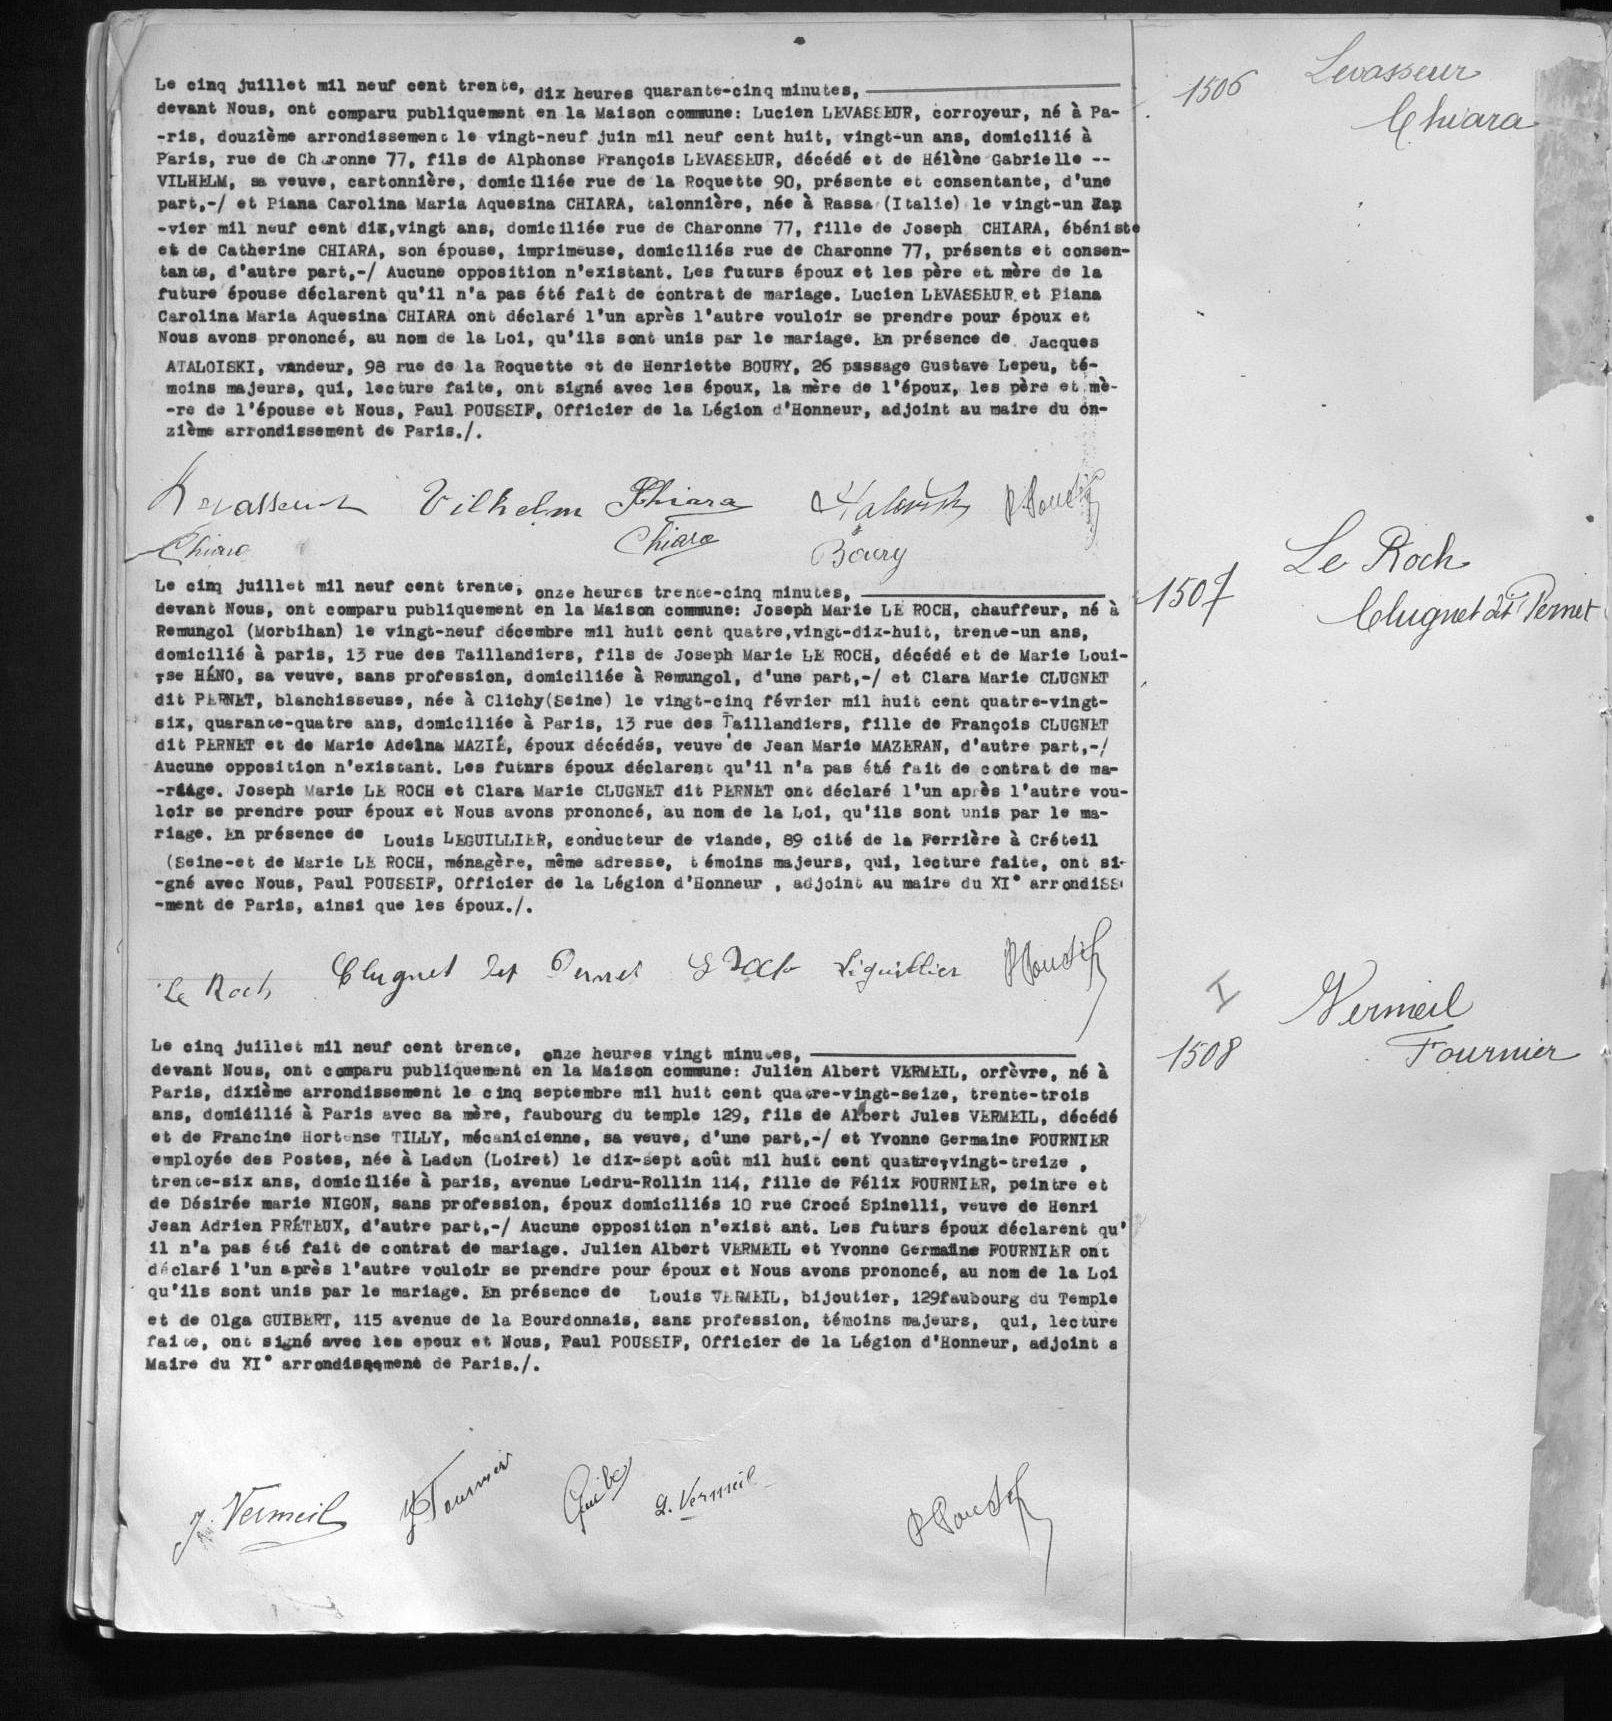Le cinq juillet mil neuf cent trente, dix heures quarante-cinq minutes, -
devant Nous, ont comparu en la Maison commune: Lucien LEVASSEUR, corroyeur, né à Pa-
-ris, douzième arrondissement le vingt-neuf juin mil neuf cent huit, vingt-un ans, domicilié à
Paris, rue de Charonne 77, fils de Alphonse François LEVASSEUR, décédé et de Hélène Gabrielle -
VILHELM, sa veuve, cartonnière, domiciliée rue de la Roquette 90, présente et consentante, d'une
part ,-/ et Piana Carolina Maria Aquesina CHIARA, talonnière, née à Rassa (Italie) le vingt-un Van
-vier mil neuf cent dix, vingt ans, domiciliée rue de Charonne 77, fille de Joseph CHIARA, ébéniste
et de Catherine CHIARA, son épouse, imprimeuse, domiciliés rue de Charonne 77, présents et conse-
tants, d'autre part ,-/ Aucune opposition n'existant. Les futurs époux et les père et mère de la
future épouse déclarent qu'il n'a pas été fait de contrat de mariage. Lucien LEVASSEUR et Piana
Carolina Maria Aquesina CHIARA ont déclaré l'un après l'autre vouloir se prendre pour époux et
Nous avons prononcé, au nom de la Loi, qu'ils sont unis par le mariage. En présence de Jacques
ATALOISKI, vandeur, 98 rue de la Roquette et de Henriette BOURY, 26 passage Gustave Lepeu, té-
moins majeurs, qui, lecture faite, ont signé avec les époux, la mère de l'époux, les père et mè-
-re de l'épouse de Nous, Paul POUSSIF, Officier de la Légion d'Honneur, adjoint au maire du on-
zième arrondissement de Paris.
Le cinq juillet mil neuf cent trente, onze heures trente-cinq minutes ,-
devant Nous, ont comparu publiquement en la Maison commune: Joseph Marie LE ROCH, chauffeur, né à
Remungol (Morbihan) le vingt-neuf décembre mil huit cent quatre, vingt-dix-huit, trente-un ans,
domicilié à paris, 13 rue des Taillandiers, fils de Joseph Marie LE ROCH, décédé et de Marie Loui-
-se HENO, sa veuve, sans profession, domiciliée à Remungol, d'une part ,-/ et Clara Marie CLUGNET
dit PERNET, blanchisseuse, né à Clichy (Seine) le vingt-cinq février mil huit cent quatre-vingt-
six, quarante-quatre ans, domiciliée à Paris, 13 rue des Taillandiers, fille de François CLUGNET
dit PERNET et de Marie Adelna MAZIE, époux décédés, veuve de Jean Marie MAZERAN, d'autre part ,-/
Aucune opposition n'existant. Les futurs époux déclarent qu'il n'a pas été fait de contrat de ma-
-raage. Joseph Marie LE ROCH et Clara Marie CLUGNET dit PERNET ont déclaré l'un après l'autre vou-
loir se prendre pour époux et Nous avons prononcé, au nom de la Loi, qu'ils sont unis par le ma-
-riage. En présence de Louis LEGUILLIER, conducteur de viande, 89 cité de la Ferrière à créteil
(Seine-et de Marie LE ROCH, ménagère, même adresse, témoins majeurs, qui, lecture faite, ont si-
-gné avec Nous, Paul POUSSIF, Officier de la Légion d'Honneur, adjoint au maire du XI ° arrondiSS
-ment de Paris, ainsi que les époux ./.
Le cinq juillet mil neuf cent trente, onze heures vingt minutes, -
devant Nous, ont comparu publiquement en la Maison commune: Julien Albert VERMEIL, orfèvre, né à
Paris, dixième arrondissement le cinq septembre mil huit cent quatre-vingt-seize, trente-trois
ans, domicilié à Paris avec sa mère, aubourg du temple 129, fils de Albert Jules VERMEIL, décédé
et de Francine Hortense TILLY, mécanicienne, sa veuve, d'une part ,-/ et Yvonne Germaine FOURNIER
employée des Postes, née à Ladon (Loiret) le dix-sept août mil huit cent quatre-vingt-treize,
trente-six ans, domiciliée à paris, avenue Ledru-Rollin 114, fille de Félix FOURNIER, peintre et
de Désiré marie NIGON, sans profession, époux domiciliés 10 rue Crocé Spinelli, veuve de Henri
Jean Adrien PRETEUX, d'autre part ,-/ Aucune opposition n'existant. Les futurs époux déclarent qu'
il n'a pas été fait de contrat de mariage. Julien Albert VERMEIL et Yvonne Germaine FOURNIER ont
déclaré l'un après l'autre vouloir se prendre pour époux et Nous avons prononcé, au nom de la Loi
qu'ils sont unis par le mariage. En présence de Louis VERMEIL, bijoutier, 129 faubourg du Temple
et de Olga GUILBERT, 115 avenue de la Bourdonnais, sans profession, témoins majeurs, qui, lecture
faite, ont signé avec les epoux et Nous, Paul POUSSIF, Officier de la Légion d'Honneur, adjoint a
Maire du XI ° arrondissement de Paris ./.
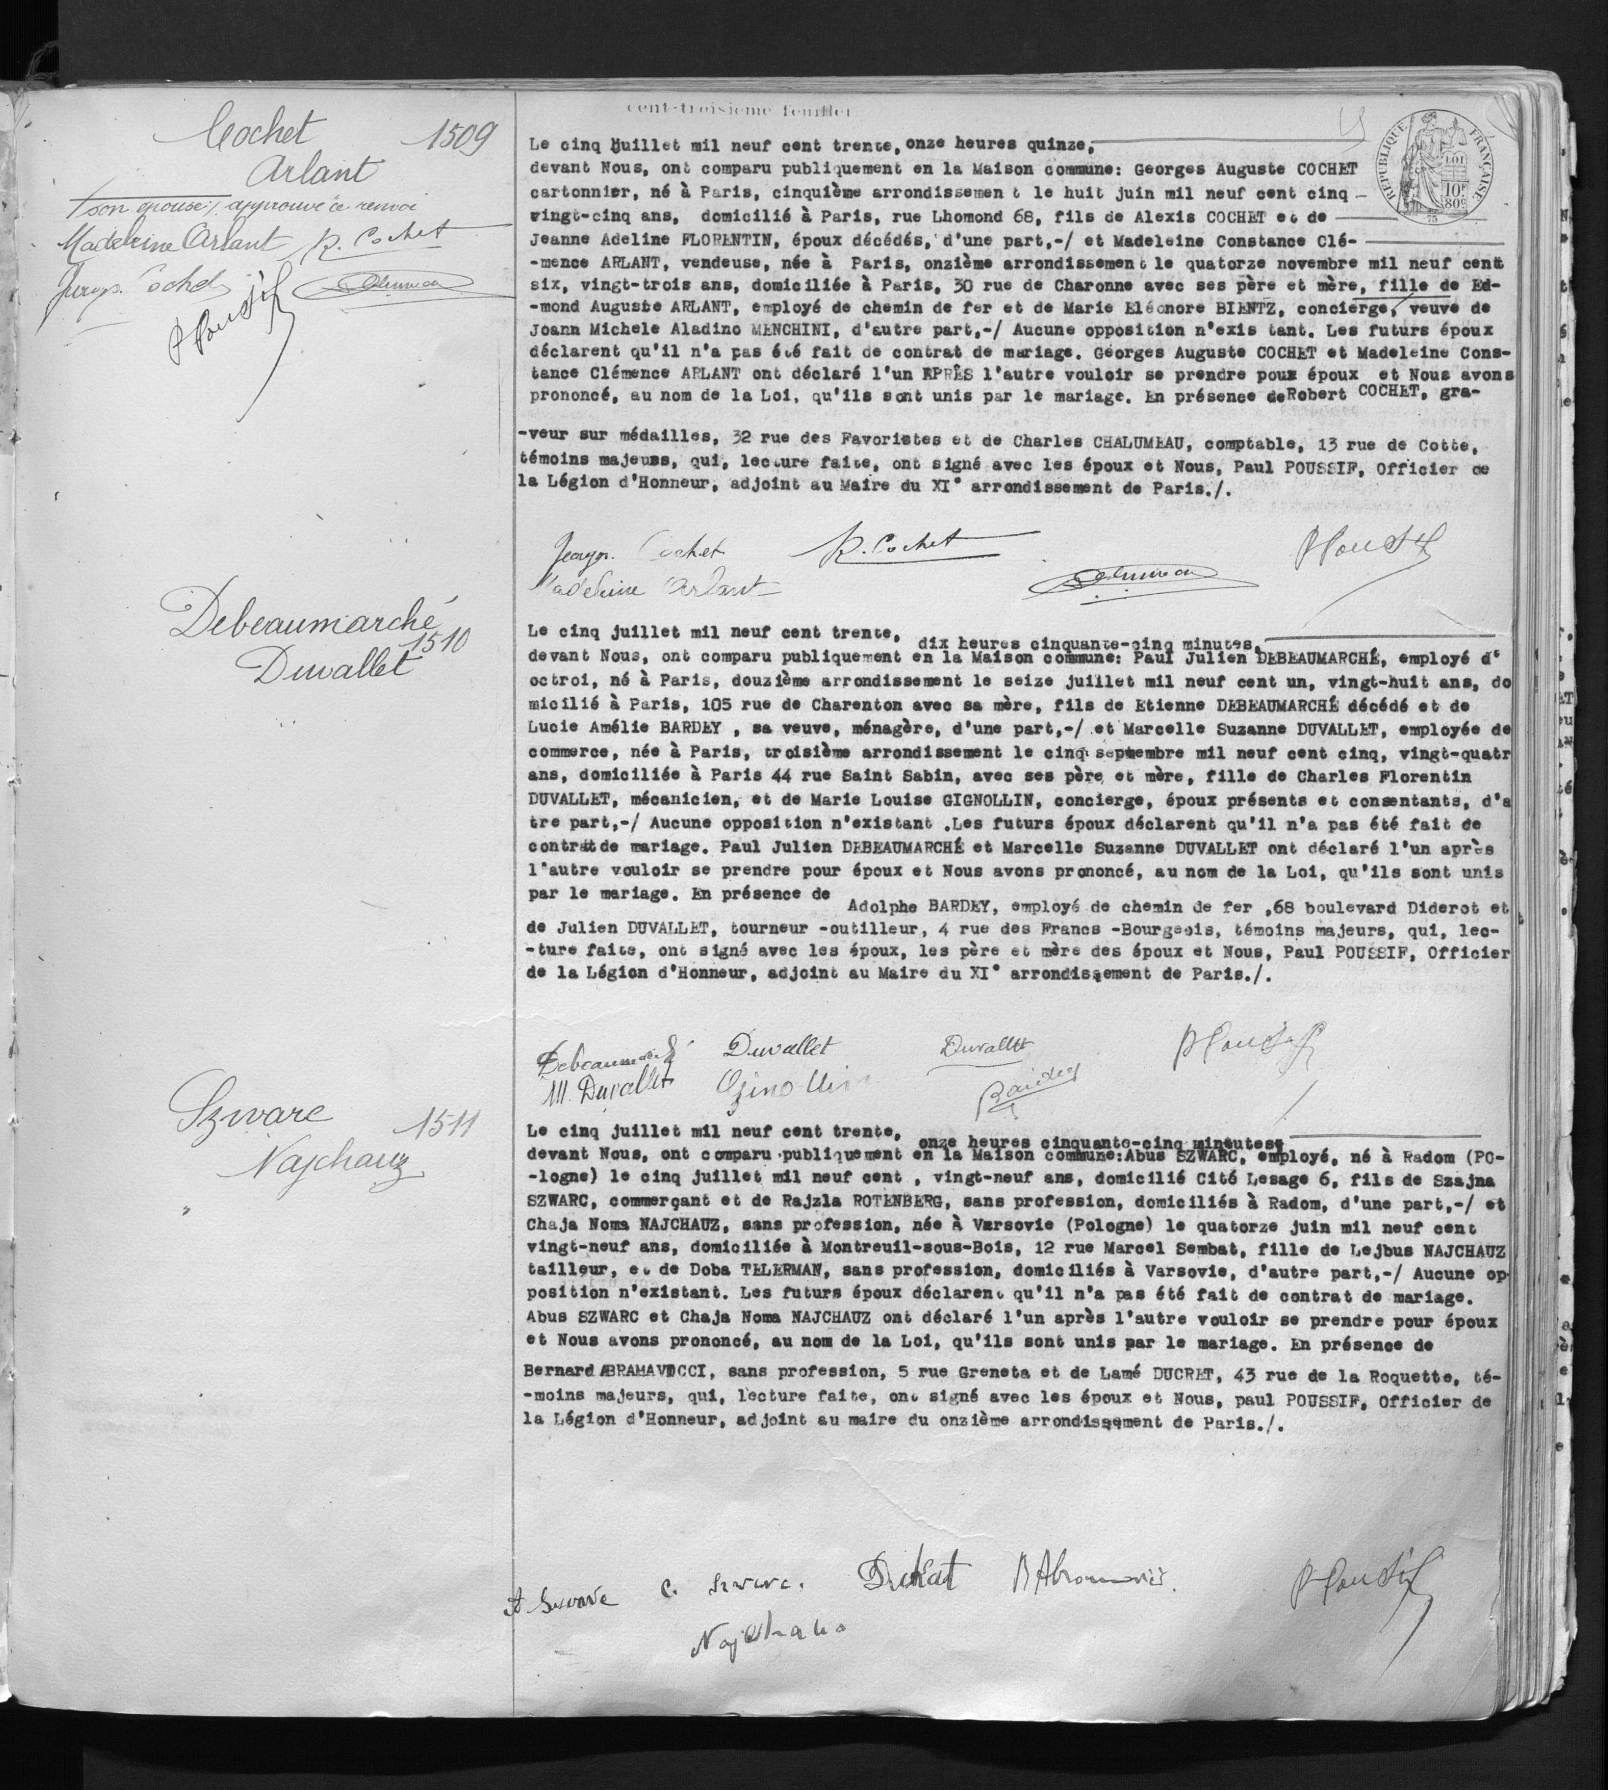Le cinq juillet mil neuf cent trente, onze heures quinze, -
devant Nous, ont comparu publiquement en la Maison commune: Georges Auguste COCHET
cartonnier, né à Paris, cinquième arrondissemen t le huit juin mil neuf cent cinq-
vingt-cinq ans, domicilié à Paris, rue Lhomond 68, fils de Alexis COCHET et de -
Jeanne Adeline FLORENTIN, époux décédés, d'une part ,-/ et Madeleine Constance Clé -
-mence ARLANT, vendeuse, née à Paris, onzième arrondissemen t le quatorze novembre mil neuf cent
six, vingt-trois ans, domiciliée à Paris, 30 rue de Charonne avec ses père et mère, fille de Ed-
-mond Auguste ARLANT, employé de chemin de fer et de Marie Eléonore BIENTZ, concierge, veuve de
Joann Michel Aladino MENCHINI, d'autre part ,-/ Aucune opposition n'exis tant. Les futurs époux
déclarent qu'il n'a pas été fait de contrat de mariage. Georges Auguste COCHET et Madelein Cons-
tance Clémence ARLANT ont déclaré l'un APRES l'autre vouloir se prendre pour époux et Nous avons
prononcé, au nom de la Loi, qu'ils sont unis par le mariage. En présence de Robert COCHET, gra-
-veur sur médailles, 32 rue des Favoristes et de Charles CHALUMEAU, comptable, 13 rue de Cotte,
témoins majeurs, qui, lecture faite, ont signé avec les époux et Nous, Paul POUSSIF, Officier ce
la Légion d'Honneur, adjoint au Maire du XI ° arrondissement de Paris.
Le cinq juillet mil neuf cent trente, dix heures cinquante-cinq minutes ,-
devant Nous, ont comparu publiquement en la Maison commune: Paul Julien DEBEAUMARCHE, employé d'
octroi, né à Paris, douzième arrondissement le seize juillet mil neuf cent un, vingt-huit ans, do
micilié à Paris, 105 rue de Charenton avec sa mère, fils de Etienne DEBEAUMARCHE décédé et de
Lucie Amélie BARDEY, sa veuve, ménagère, d'une part ,-/ et Marcelle Suzanne DUVALLET, employée de
commerce, née à Paris, troisième arrondissement le cinq septembre mil neuf cent cinq, vingt-quatr
ans, domiciliée à Paris 44 rue Saint Sabin, avec ses père et mère, fille de Charles Florentin
DUVALLET, mécanicien, et de Marie Louis GIGNOLLIN, concierge, époux présents et consentants, d'au
tre part ,-/ Aucune opposition n'existant. Les futurs époux déclarent qu'il n'a pas été fait de
contrat de mariage. Paul Julien DEBAUMARCHE et Mercelle Suzanne DUVALLET ont délcaré l'un après
l'autre vouloir se prendre pour époux et Nous avons prononcé, au nom de la Loi, qu'ils sont unis
par le mariage. En présence de Adolphe BARDEY, employé de chemin de fer, 68 boulevard Diderot et
de Julien DUVALLET, tourneur-outilleur, 4 rue des Francs-Bourgeois, témoins majeurs, qui, lec-
-ture faite, ont signé avec les époux, les père et mère des époux et Nous, Paul POUSSIF, Officier
de la Légion d'Honneur, adjoint au Maire du XI ° arrondissement de Paris ./.
Le cinq juillet mil neuf cent trente, onze heures cinquante-cinq minutes, -
devant Nous, ont comparu publiquement en la Maison commune: Abus SZWARC, employé, né à Radom (PO-
logne) le cinq juillet mil neuf cent, vingt-neuf ans, domicilié Cité Lesage 6, fils de Szajna
SZWARC, commerçant et de Rajzla ROTENBERG, sans profession, domicilis à Radom, d'une part ,-/ et
Chaja Noms NAJCHAUZ, sans profession, née à Varsovie (Pologne) le quatorze juin mil neuf cent
vingt-neuf ans, domiciliée à Montreuil-sous-Bois, 12 rue Marcel Sembat, fille de Lebjus NAJCHAUZ
tailleur, e de Doba TELLERMAN, sans profession, domicilis à Varsovie, d'autre part ,-/ Aucune op-
position n'existant. Les futurs époux déclaren qu'il n'a pas été fait de contrat de mariage.
Abus SZWARC et Chaja Noma NAJCHAUZ ont déclaré l'un après l'autre vouloir se prendre pour époux
et Nous avons prononcé, au nom de la Loi, qu'ils sont unis par le mariage. En présence de
Bernard ABRAMAVCCI, sans profession, 5 rue Greneta et de Lamé DUCRET, 43 rue de la Roquette, té-
-moins majeurs, qui, lecture faite, ont signé avec les époux et Nous, paul POUSSIF, Officier de
la Légion d'Honneur, adjoint au maire du onzième arrondissement de Paris ./.